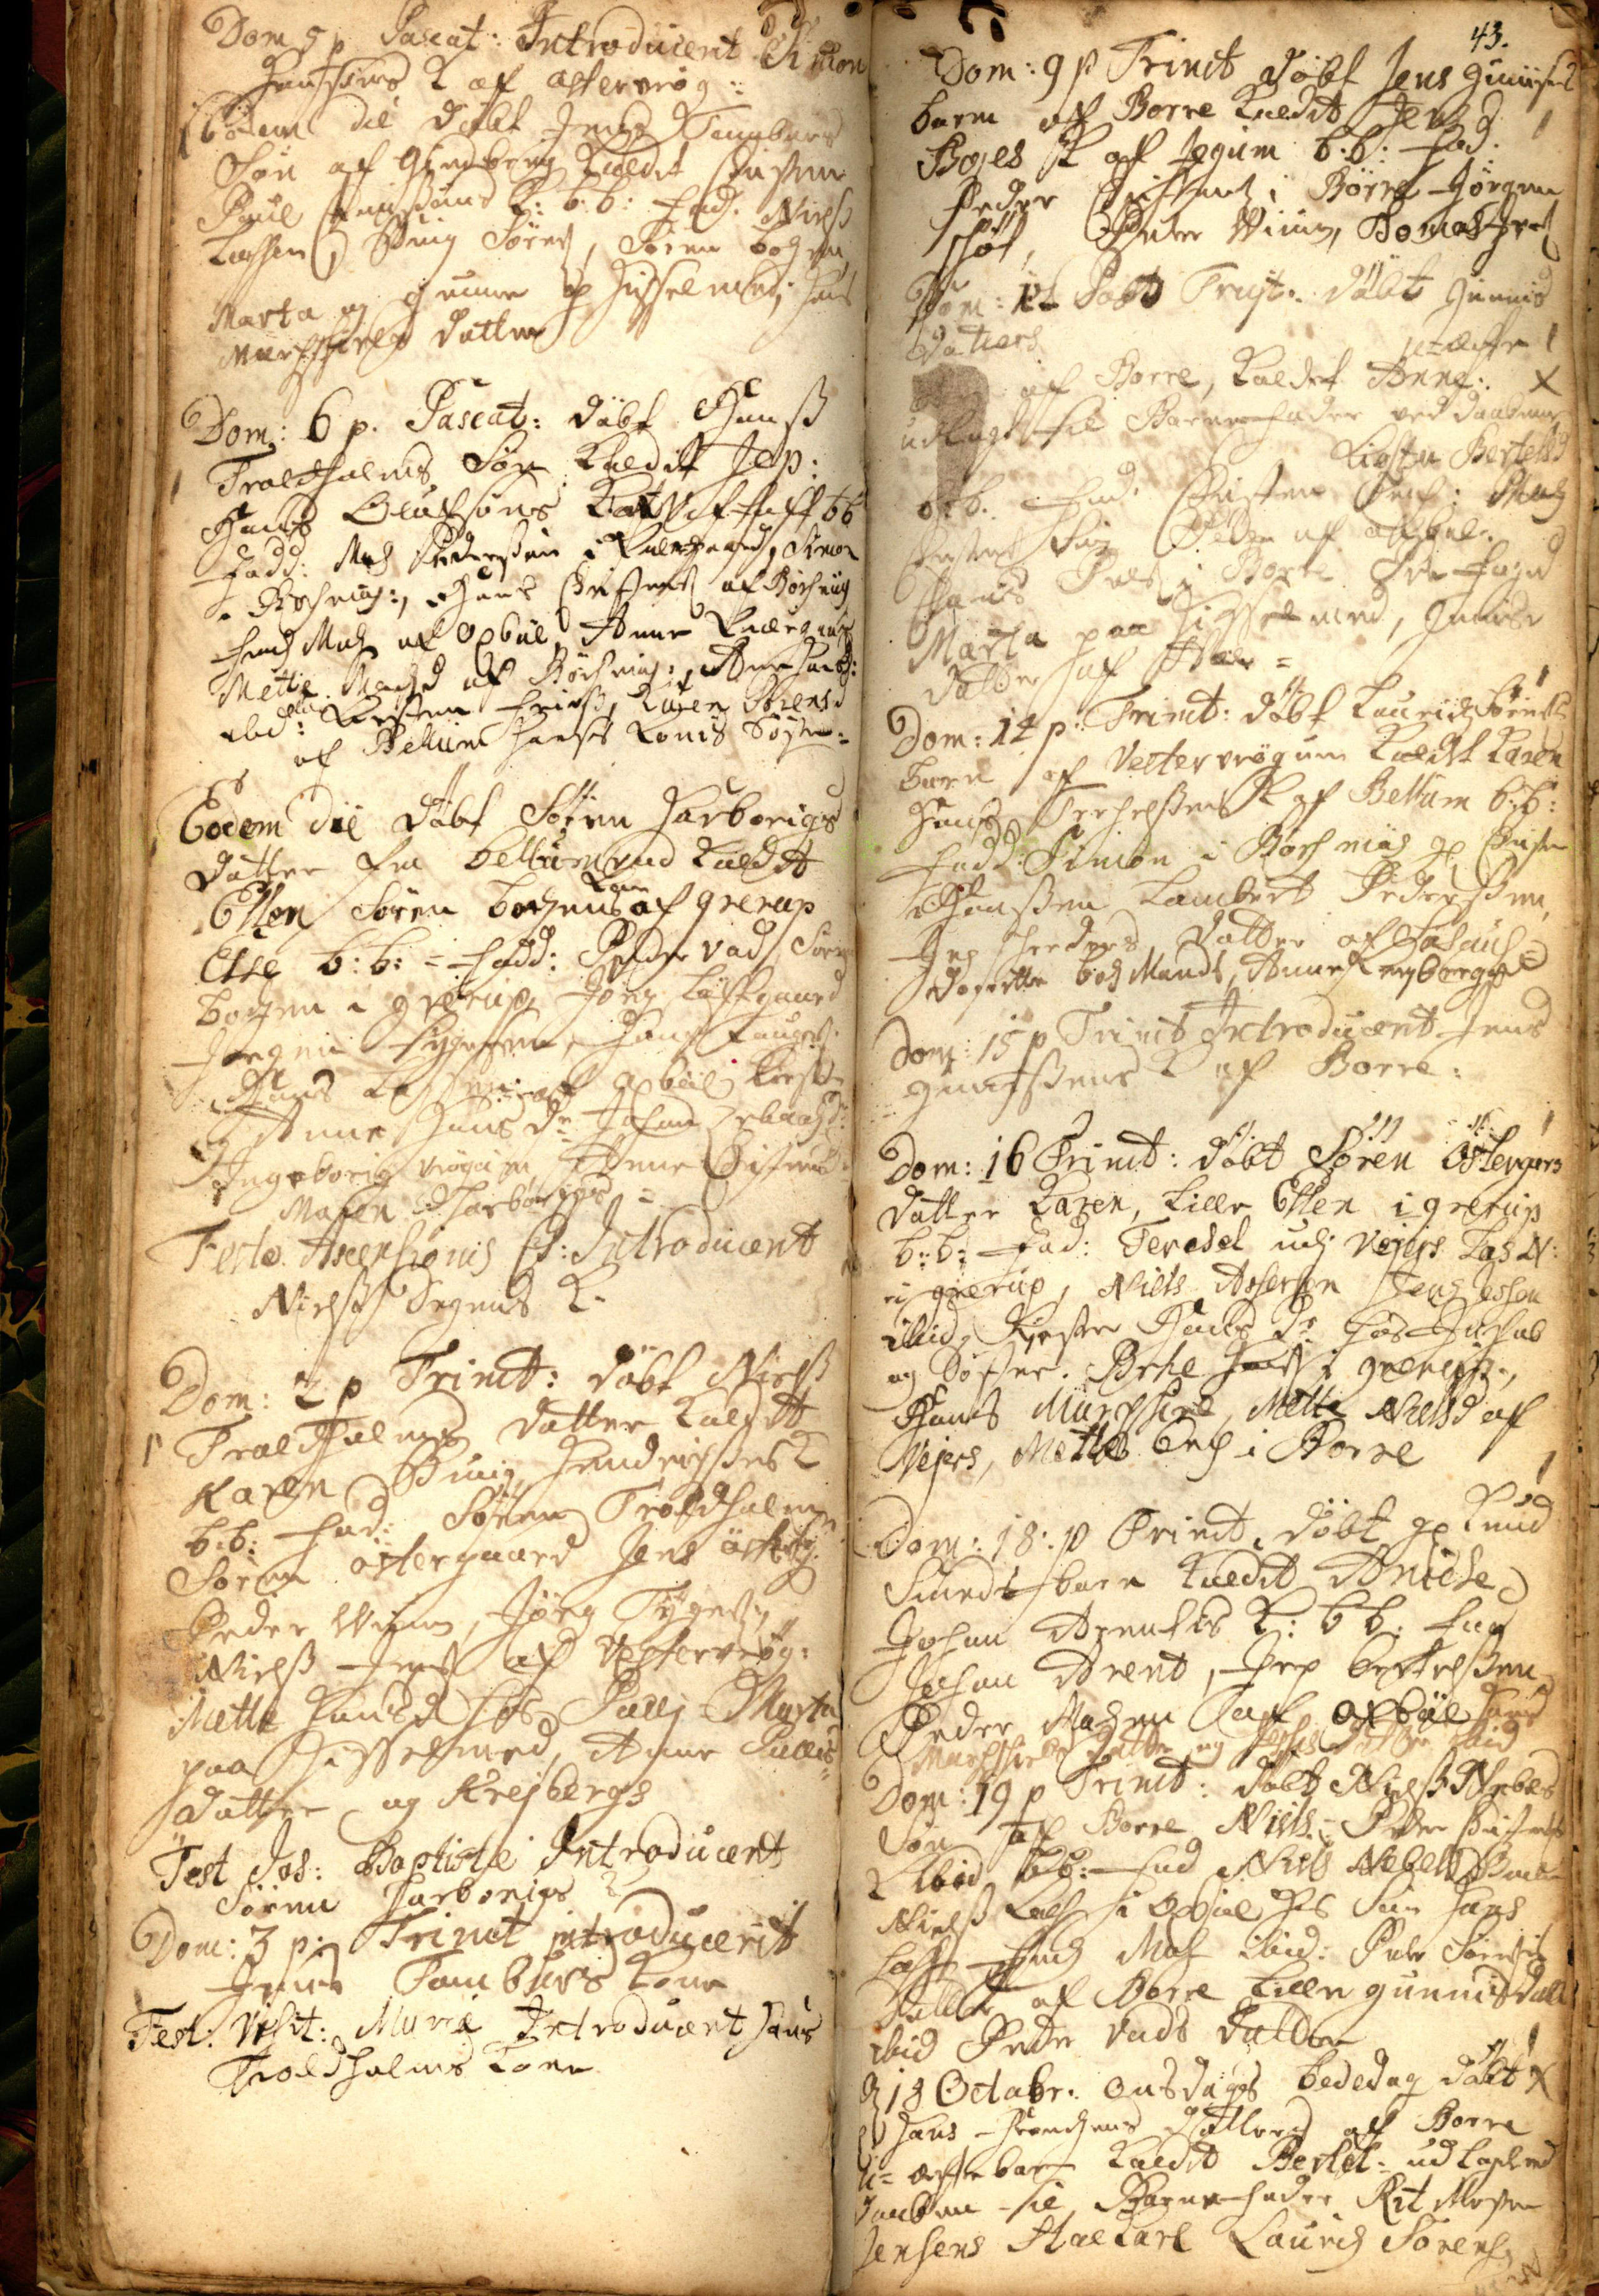Dom 5 p Pascat: Introducerit Simon
Hansøns K af Østervrøg:
Eodem Die døbt Jens Tamburs
Søn af Giedberig kaldet Christen
Poul Christensøns K: b: b: Fad. Niels
Lassen, Bunj Sørens, Søren Bodzen
Marta og Gunner p Hisselmed; Hans
Marchschiels Datter
Dom: 6 p. Pascat: døbt Hans
Troldholms Søn kaldit Jep:
Hans Olufsøns K. af Viftoft bb
Fadd: Madz Pedersøn i Kiergaard, Simon
i Børsmøs:, Hans Christensen af Børsmøs
Frandz Madz:, af Oxbøl, Anne Kiergaard,
Mette Madzd af Børsmøs:, Anne Hansd:
ibid: Kirsten Friis, Karen Sorensd##
af Bellum Hanses Konis Søster=
Eodem Die døbt Søren Harborigs
Datter fra Bellumvad kaldet
Kone
Ellen Søren Bodzens af Grerup
Else b: b: Fadd: Peder Vad Søren
Bodzen i Grerup, Jørg Løftgaard
Jørgen Tygesen, Hans ##.
Hans Lassen## af Oxbøl Kirsten
og Anne Hansdr Johanne Zebæuzdr
Ingeborig Vrøgum, Anne Christensdr
Maren Harborigs=
Festo Ascensionis Ch: Introducerit
Niels Degens K.
Dom: 2 p Trinit: døbt Niels
Troldholms Datter kaldet
Karen Buni Hendichsens K
b:b: Fad: Søren Troldholm
Søren Østergaard, Jens Østerg.
Peder Wium, Jørgen Tygesen,
Niels Jens af Vestervrøg:
Mette Hansd hos Pallj= Marta
paa Hisselmed, Anne Pallis
datter og Krejbergs
Fest Joh: Baptistæ Introducerit
Søren Harborigs K
Dom: 3 p: Trinit introducerit
Jens Tamburs Kone
Fest: Visit: Maræ Introducerit Hans
Troldholms Kone
43.
Dom: 9 p Trinit døbt Jens Gunnisens
Barn af Borre kaldit Jens.
Bojes K af Jegum b:b: fad.
Peder Christens i Borre Jørgen
Schøt, Peder Wium, Thomas Iver##s
Dom: 12 Post Trinjt: døbt Gunnis
Datters
u-æchte
##
barn## af Borre, kaldet Anne.
udlagt til Barnefader ved Daaben
Kirsten Bertelsd
b:b: Fad: Christen ##: Madz
Christensens Søn Peder af Oxbøl.
Claus Peders i Borre, Peder Foged
Marta paa Hisselmed, Gunnis##
Datter af ##=
Dom: 14 p: Trinit: døbt Lauridtz ##
Barn af Vestervrøgum kaldet Karen.
Hans Terchelsens K af Bellum b:b:
Fadd: Simon i Børsmøs gl Christen
Hansen Lambert Pedersen,
Jep Schredrs Datter af Søhuus
Dorrette Bodz Mands, Anne Krejbergs=
Dom: 15 p Trinit Introducerit Jens
Gunnisens K af Borre:
Dom: 16 Trinit: døbt Søren Østergaars
Datter Karen, Lille Ellen i Grerup
b:b: Fad: Terchel udi Vejers, Las N:
i Grerup, Niels Assersen Jens Jessen
ibid, Kirsten Hansdr hos Jachob
og Søster. Bertel Hans i Grerup,
Hans Marchschiel, Mette Nielsd af
Vejers, Mette Brich i Borre
Dom:18: p Trinit, døbt gl Knud
Smeds Barn kaldit Aniche
Johan Arentis K: bb. Fad
Johan Arent, Jep Bertelsen,
Peder Madsen af Oxbøl Hans
Marchschiels Datter og Jessis Datter ibid
Dom: 19 p Trinit: døbt Niels Nebels
Søn af Borre Niels. Peder Christens
K ibid bb: Fad Niels Nebels Broder
Niels Las## i Oxbøl, Hs Søn Hans
Las ## Mads ibid: Peder Sørens
Datter af Borre, Lille Gunnis Datter
ibid Peder Vads Datter
X
D 18 Octobr. Onsdags Bededag døbt
Hans Frandzens Datters af Borre
u=ægte Barn kaldet Bertel= udlagt ved
Daaben til Barnefader Rit Mester
Jensens Stalkarl Lauridz Sørens
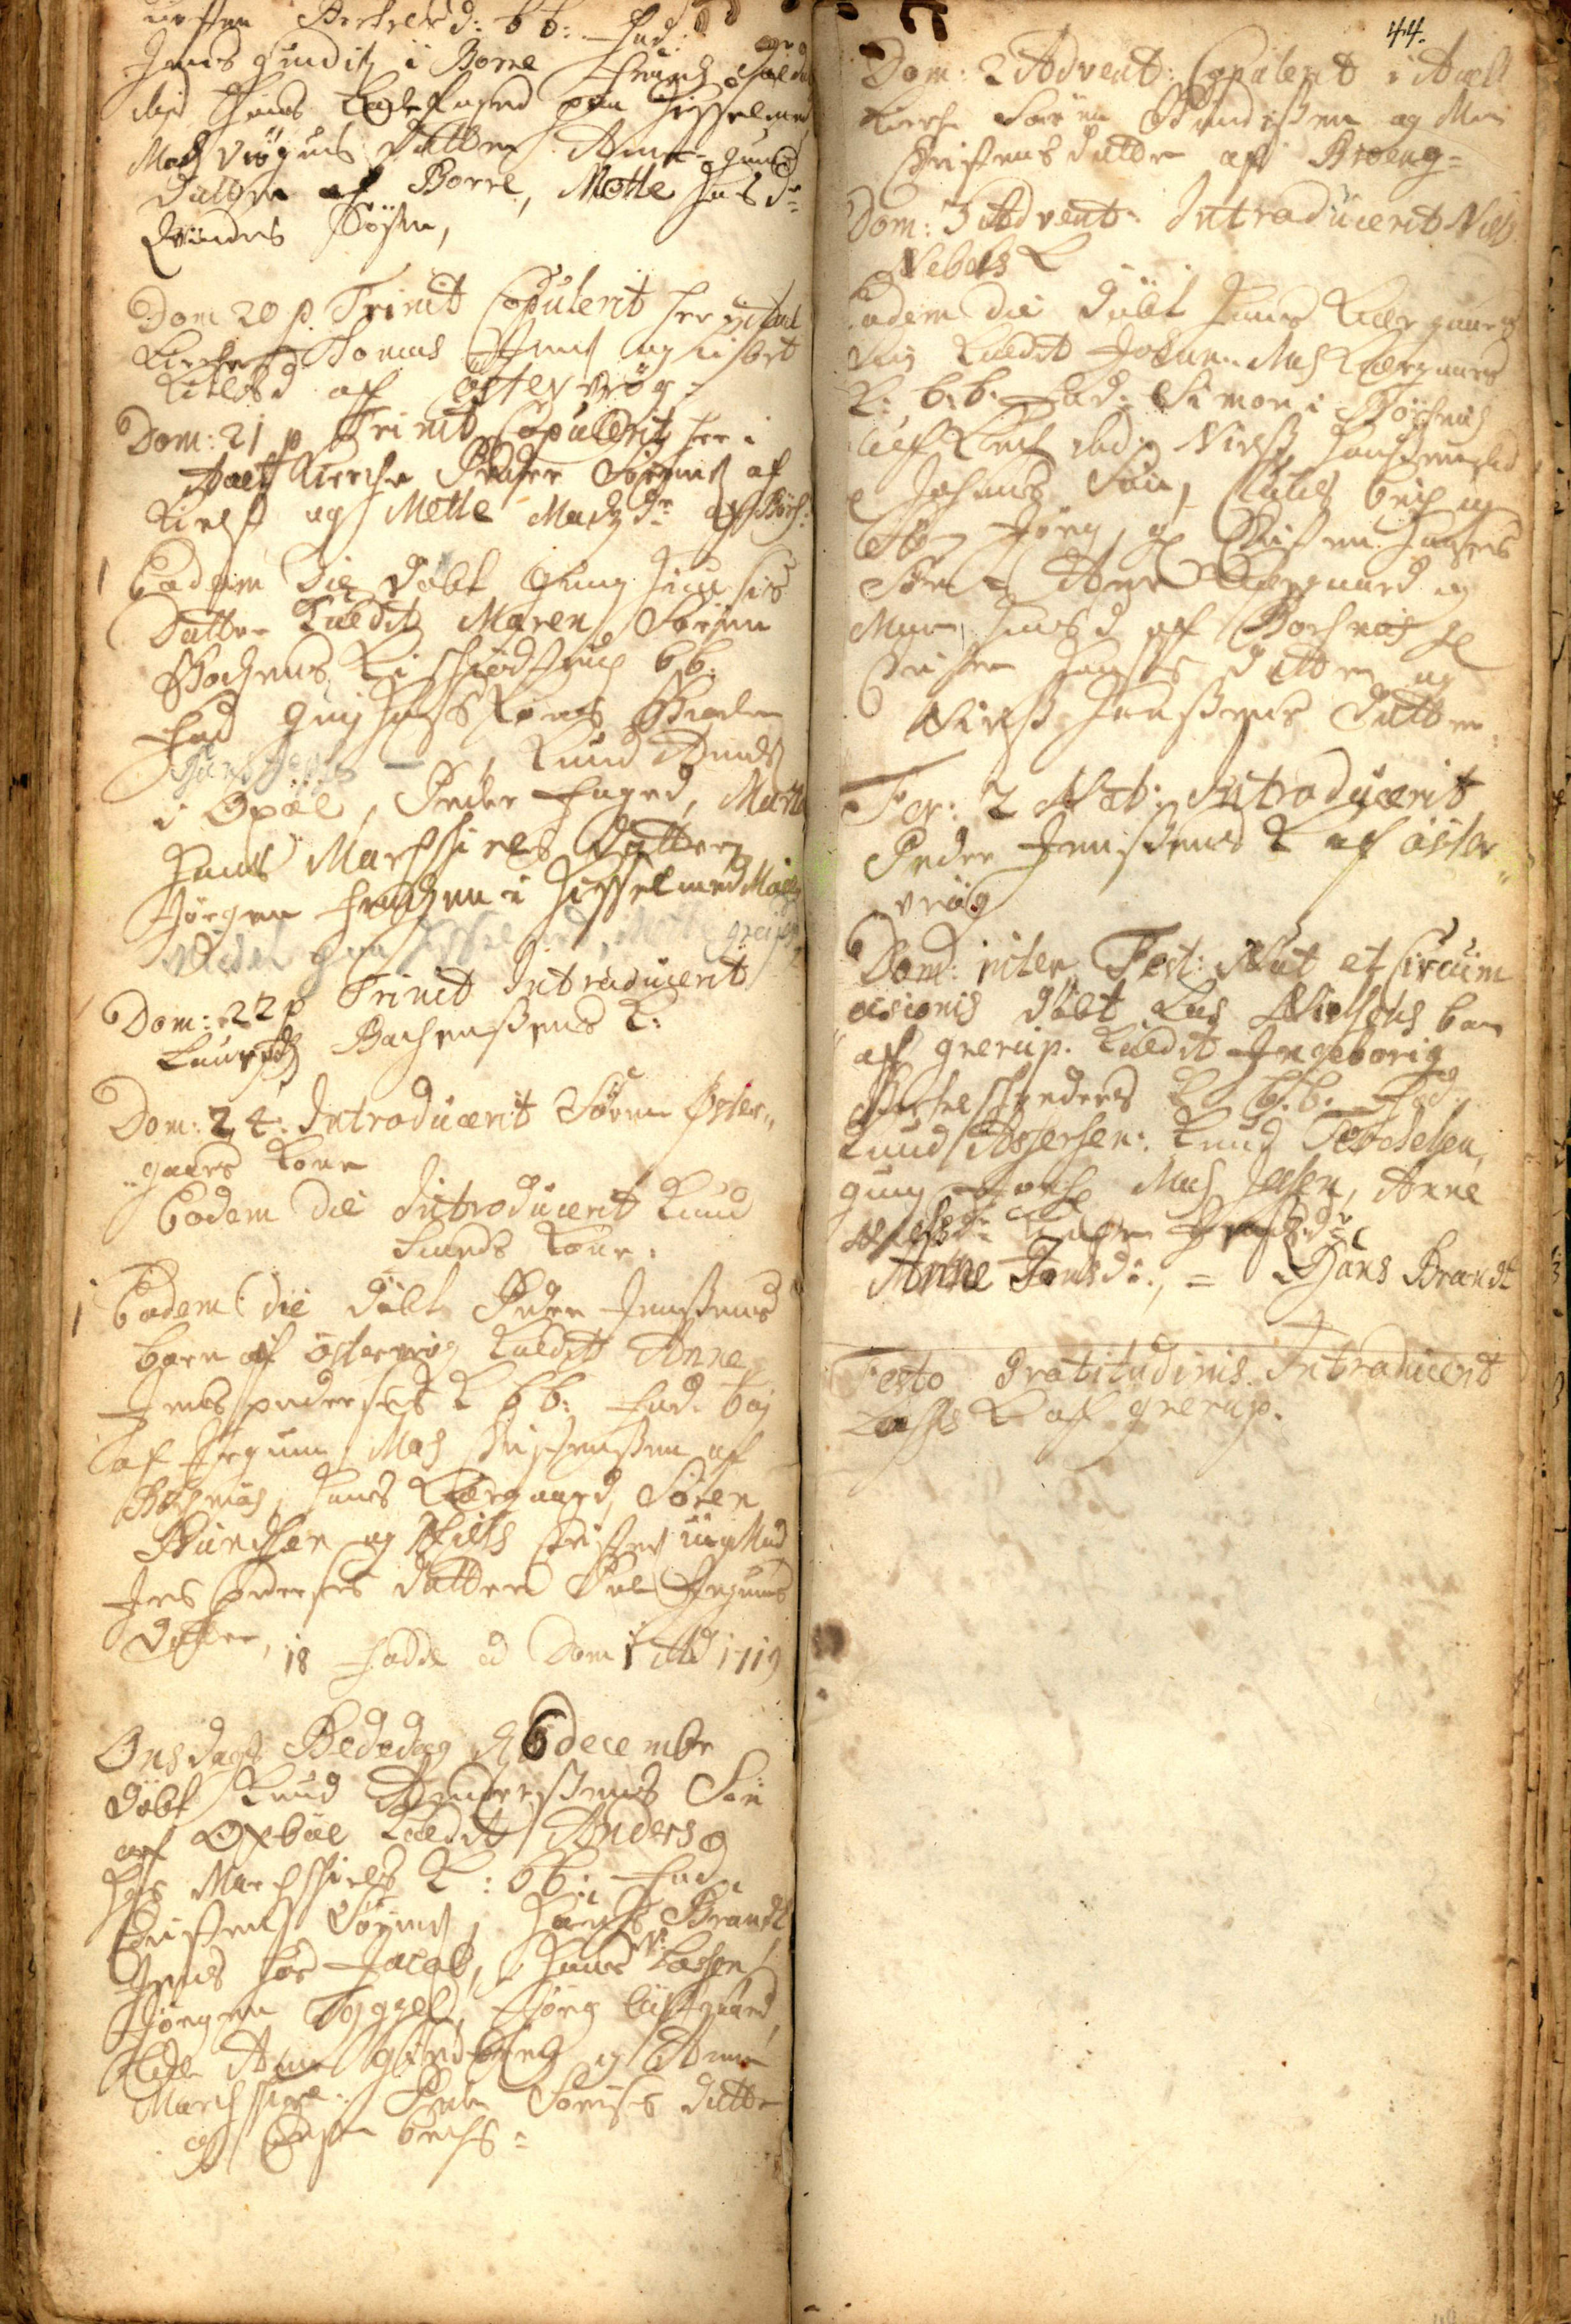Kirsten Bertelsd: bb. Fad:
Jens Gundis i Borre Frandz Soldat
ibid Hans Ladefoged paa Hisselmed
Madz Vrøgums Datter, Anne Gunnis
datter af Borre, Mette Hansdr
Qvindens Søster,
Dom 20 p. Trinit Copulerit her i Aael
Kirche Thomas Jens og Lisbet
Kielsd af Østervrøg=
Dom: 21 p Trinit Copulerit her i
Aael Kirche Peder Sørens af
Kielst og Mette Madzdr af Børs:
Eodem Die Døbt Gunj Hansis
Datter kaldit Maren Søren
Bodzens K i Schiødstrup bb:
Fad: Gunj Hans Kones Broder
##
Knud An##
i Oxbøl, Peder Foged, Marta
Hans Marchschiels Datter
Jørgen Frandzen i Hisselmed Mølle,
## paa Hisselmed, Mette Greijs##
Dom: 22 p Trinit Introducerit
Lauridz Bachensens K:
Dom: 24 Introducerit Søren Øster¬
gaars Kone
Eodem Die Introducerit Knud
Smeds Kone
Eodem Die døbt Peder Jensens
Barn af Østrevrøg kaldet Anne
Jens Pedersens K bb: Fad: Boj
af Jegum Madz Christensen af
Børsmøs, Hans Kiærgaard, Søren
Bundsen og Niels Christensen ung Mand##
Jes Pedersens Datter Peder Jegums
Datter. 18 Fødde udi Dom## Ao 1719
Onsdags Bededag d 6 Decembr
døbt Knud Andersens Søn
af Oxbøl kaldit Anders
Hans Marchschiels K: bb: Fad
Christen Søren, Hans Brandt
Jens hos Jacob, Hans Lassen,
Jørgen Tyges, Jørg Løftgaard,
Lille## Anne Giedbereg og Anne
Marchschiel: Peder Sørens Datter
og ## Brichs
44
Dom: 2 Advent: Copulerit i Aael
Kirche Søren Bundissen og Maren
Christensdatter af Broeng=
Dom: 3 Advent: Introducerit Niels
Nebels K
Eodem Die døbt Hans Kiærgaards
Søn kaldit Johan: Madz Kiærgaars
K: b:b. Fad: Simon i Børsmøs
Oluf Knuds ibid: Niels Hans ##
Johans Søn, Claus Brich og
## Jørg, gl Christen Hansens
Søn, Anne Kiærgaard og
Maren Hansd af Børsmøs gl
Christen Hans Datter og
Niels Hansens Datter
Fer: 2 Nat: Introducerit
Peder Jenssens K af Øster¬
vrøg
Dom: inter Fest: Nat et Circum
cisionis døbt Las Nielsens Barn
af Grerup. kaldit Ingeborig
Bertel Schreders K b.b. Fad:
Knud Assersen: Knud Terchelsen,
Gunj ## Madz Jessen, Anne
Nielsdr Kirsten## Fra##dr
Anne Jensdr,=  Hans Brandt
Festo Prætitudinis## Introducerit
Lassis K af Grerup